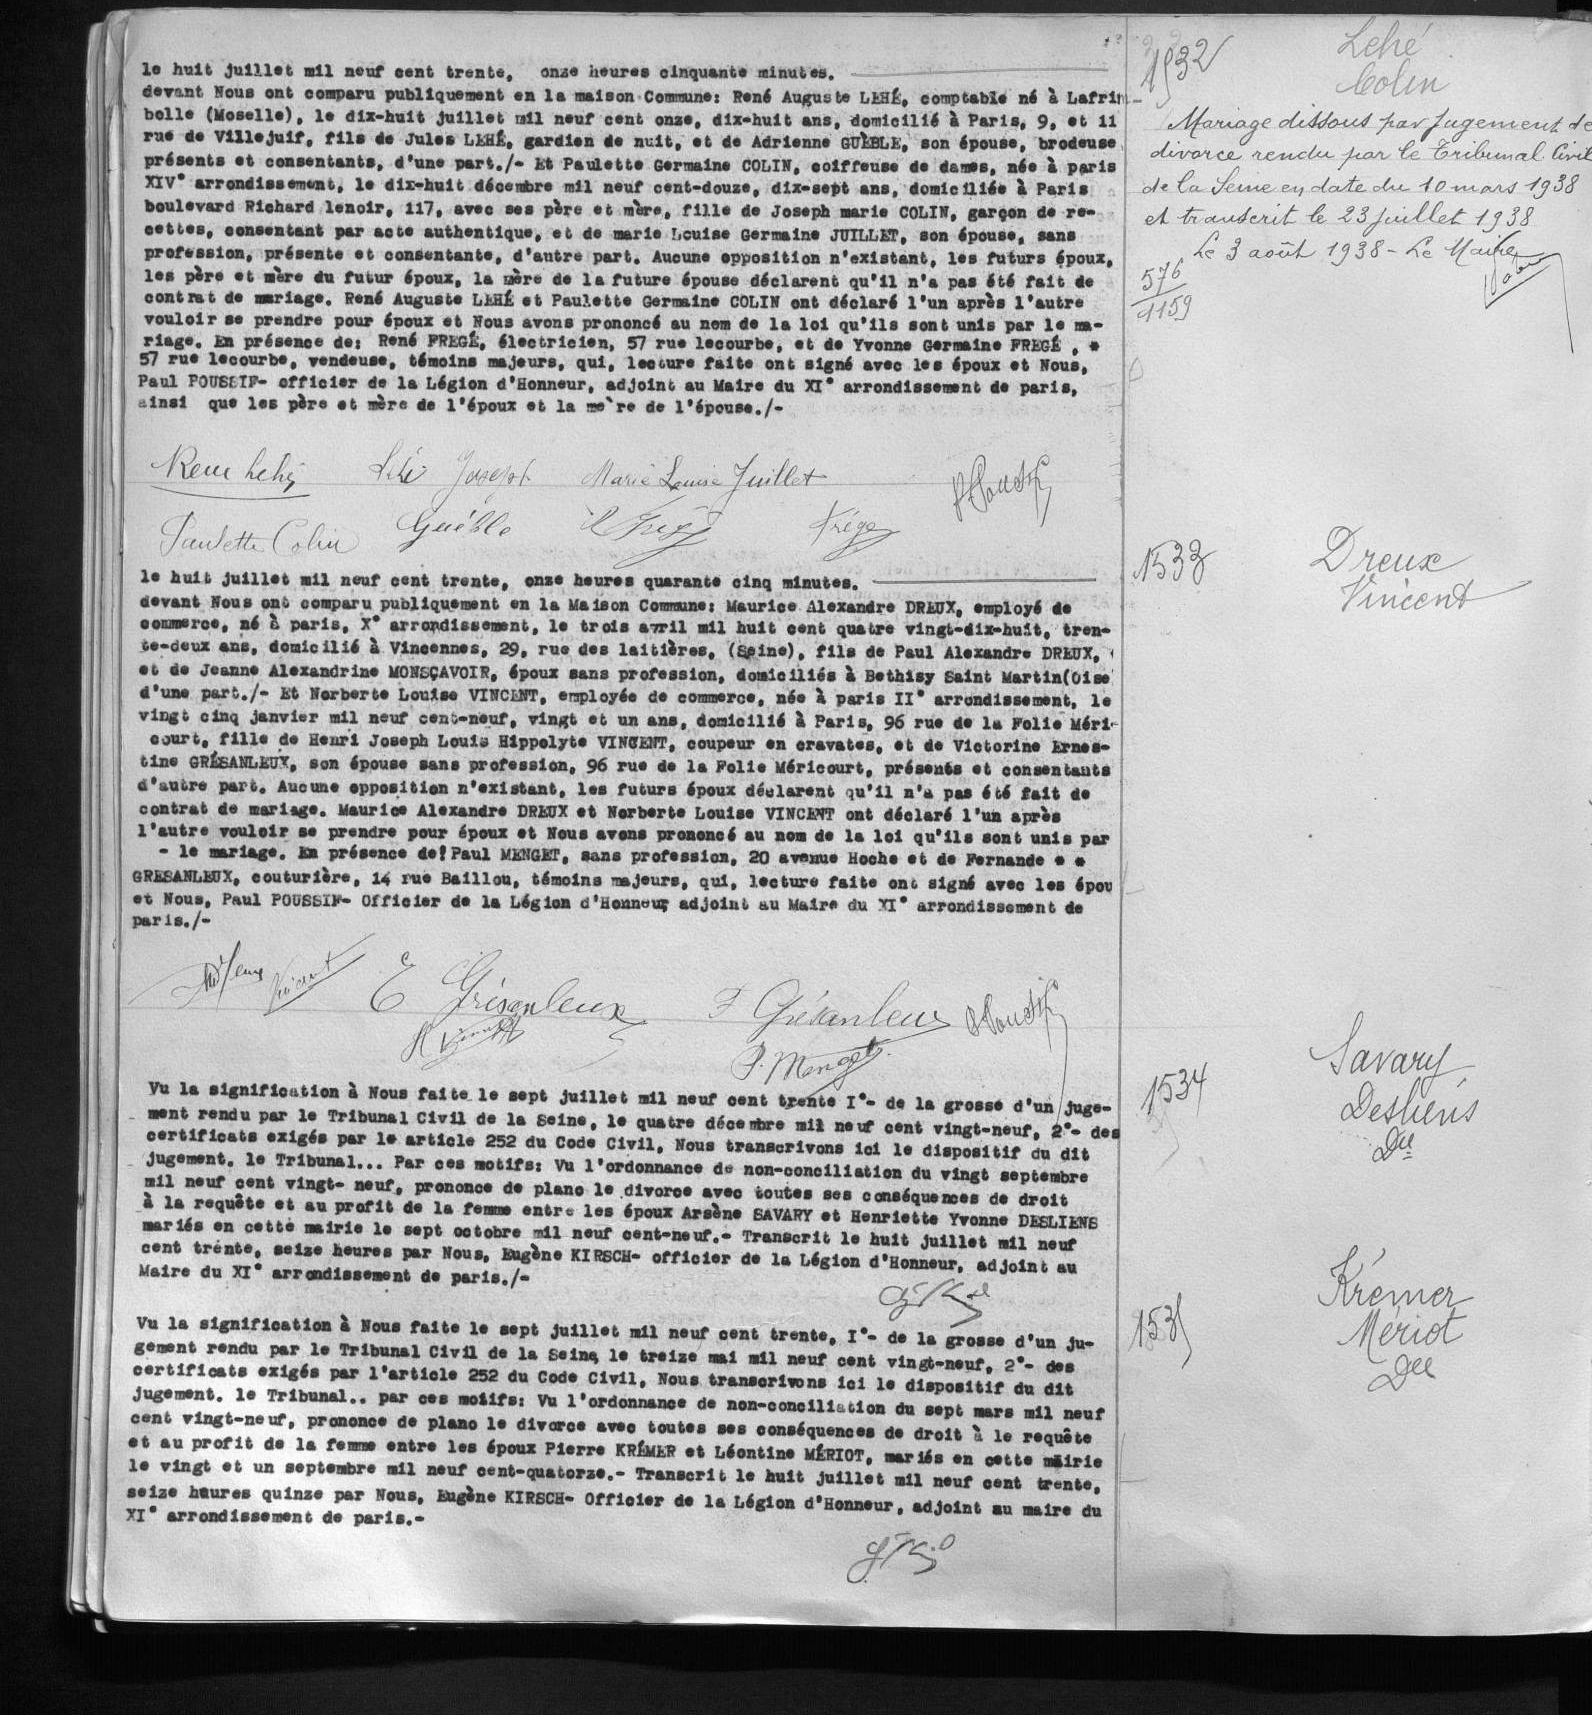le huit juillet mil neuf cent trente, onze heures cinquante minutes .-
devant Nous ont comparu publiquement en la maison commune: René Auguste LEHE, comptable né à Lafrin
belle (Moselle), le dix-huit juillet mil neuf cent onze, dix-huit ans, domicilié à Paris, 9, et 11
rue de Villejuif, fils de Jules LEHE, gardien de nuit, et de Adrienne GUEBLE, son épouse, brodeur
présents et consentants, d'une part ./-Et Paulette Germaine COLIN, coiffeuse de dames, née à paris
XIV ° arrondissement, le dix-huit décembre mil neuf cent-douze, dix-sept ans, domiciliée à Paris
boulevard Richard Lenoir, 117, avec ses père et mère, fille de de Joseph marie COLIN, garçon de re-
cettes, consentant par acte authentique, et de marie Louise Germaine JUILLET, son épouse, sans
profession, présente et consentante, d'autre part. Aucune opposition n'existant, les futrus époux,
les père et mère du futur époux, la mère de la future épouse déclarent qu'il n'a pas été fait de
contrat de mariage. René Auguste LEHE et Paulette Germaine COLIN ont déclaré l'un après l'autre
vouloir se prendre pour époux et Nous avons pronocné au nom de la loi qu'ils sont unis par le ma-
riage. En présence de: René FREGE, électricien, 57 rue lecourbe, et de Yvonne Germaine FREGE, *
57 rue lecourbe, vendeuse, témoins majeurs, qui, lecture faite ont signé avec les époux et Nous,
Paul POUSSIF-officier de la Légion d'Honneur, adjoint au Maire du XI ° arrondissement de paris,
ainsi que les père et mère de l'époux et la mère de l'épouse ./-
le huit juillet mil neuf cent trente, onze heures quarante cinq minutes .-
devant Nous ont comparu publiquement en la Maison Commune: Maurice Alexandre DREUX, employé de
commerce, né à paris, X ° arrondissement, le trois avril mil huit cent quatre vingt-dix-huit, tren-
te-deux ans, domicilié à Vincennes, 29, rue des laitières, (Seine), fils de Paul Alexandre DREUX,
et de Jeanne Alexandrine MONSCAVOIR, époux sans profession, domiciliés à Bethisy Saint Martin (Oise
d'une part ./-Et Noberte Louise VINCENT, employée de commerce, née à paris II ° arrondissement, le
vingt cinq janvier mil neuf cent-neuf, vingt et un ans, domicilié à Paris, 96 rue de la Folie Méri)
court, fille de Henri Joseph Louis Hippolyte VINCENT, coupeur en cravates, et de Victorine Erne-
tine GRESANLEUX, son épouse sans profession, 96 rue de la Folie Méricourt, présents et consentants
d'autre part. Aucune opposition n'existant, les futurs époux déclarent qu'il n'a pas été fait de
contrat de mariage. Maurice Alexandre DREUX et Norbert Louise VINCENT ont déclaré l'un après
l'autre vouloir se prendre pour époux et Nous avons prononcé au nom de la loi qu'ils sont unis par
-le mariage. En présence de: Paul MENGET, sans profession, 20 avenue Hoche et de Fernande **
GRESANLEUX, couturière, 14 rue Baillou, témoins majeurs, qui, lecture faite ont signé avec les épou
et Nous, Paul POUSSIF-Officier de la Légion d'Honneur adjoint au Maire du XI ° arrondissement de
paris ./-
Vu la signification à Nous faite le sept juillet mil neuf cent trente I °-de la grosse d'un juge-
ment rendu par le Tribunal Civil de la Seine, le quatre décembre mil neuf cent vingt-neuf, 2°-des
certificats exigés par l * article 252 du Code Civil, Nous transcrions ici le dispositif du dit
jugement. le Tribunal... Par ces motifs: Vu l'ordonnance de non-conciliation du vingt septembre
mil neuf cent vingt-neuf, prononce de plano le divorce avec toutes ses conséquences de droit
à la requête et au profit de la femme entre les époux Arsène SAVARY et Henriette Yvonne DESLIENS
mariés en cette mairie le sept octobre mil neuf cent-neuf .-Transcrit le huit juillet mil neuf
cent trente, seize heures par Nous, Eugène KIRSCH-officier de la Légion d'Honneur, adjoint au
Maire du XI ° arrondissement de paris ./-
Vu la signification à Nous faite le sept juillet mil neuf cent trente, I °-de la grosse d'un ju-
gement rendu par le Tribunal Civil de la Seine, le treize mai mil neuf cent vingt-neuf, 2°-des
certificats exigés par l'article 252 du Code Civil, Nous transcrivons ici le dispositif du dit
jugement. le Tribunal.. par ces motifs: Vu l'ordonnance de non-conciliation du sept mars mil neuf
cent vingt-neuf, prononce de plano le divorce avec toutes ses conséquences de droit à le requête
et au profit de la femme entre les époux Pierre KREMER et Léontine MERIOT, mariés en cette mairie
le vingt et un septembre mil neuf cent-quatorze .-Transcrit le huit juillet mil neuf cent trente,
seize heures quinze par Nous, Eugène KIRSCH-Officier de la Légion d'Honneur, adjoint au maire du
XI ° arrondissement de paris.-
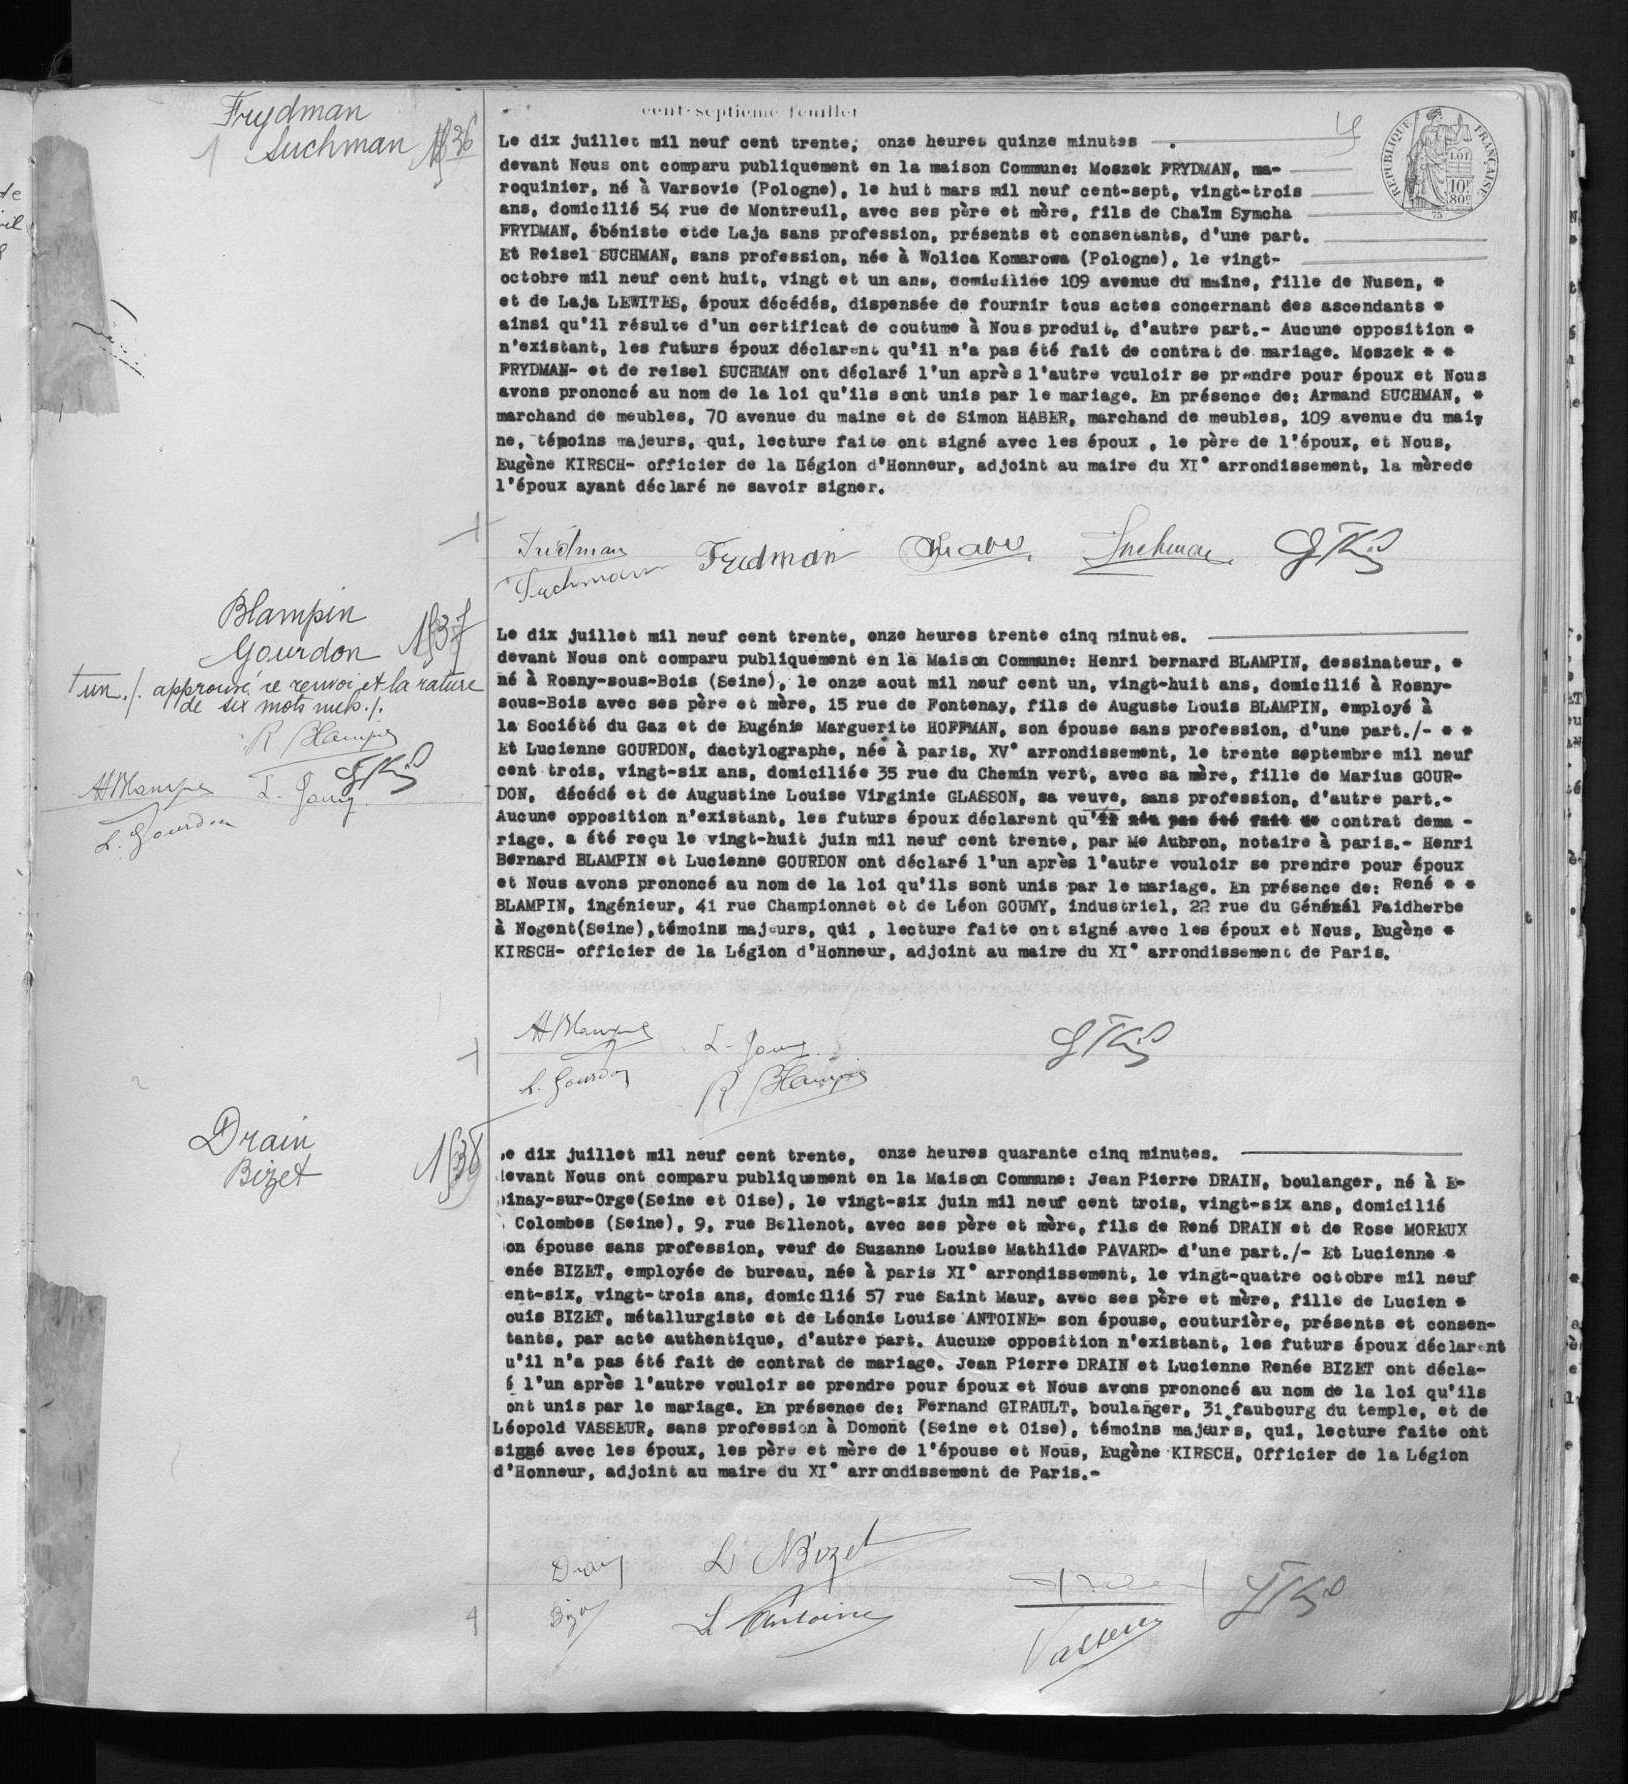Le dix juillet mil neuf cent trente: onze heures quinze minutes -
devant Nous ont comparu publiquement en la maison Commune: Moszek FRYDMAN, ma-
roquinier, né à Varsovie (Pologne), le huit mars mil neuf cent-sept, vingt-trois -
ans, domicilié 54 rue de Montreuil, avec ses père et mère, fils de Chaïm Symcha -
FRYDMAN, ébéniste et de Laja sans profession, présents et consentants, d'une part .-
Et Reisel SUCHMAN, sans profession, née à Wolica Komarowa (Pologne), le vingt -
octobre mil neuf cent huit, vingt et un ans, domiciliée 109 avenue du maine, fille de Nusen, *
et de Laja LEWITES, époux décédés, dispensée de fournir tous actes concernant des ascendants *
ainsi qu'il résulte d'un certificat de coutume à Nous produit, d'autre part .-Aucune opposition *
n'existant, les futurs époux déclarent qu'il n'a pas été fait de contrat de mariage. Moszek **
FRYDMAN-et de reisel SUCHMAN ont déclaré l'un après l'autre vouloir se prendre pour époux et Nous
avons prononcé au nom de la loi qu'ils sont unis par le mariage. En présence de: Armand SUCHMAN, *
marchand de meubles, 70 avenue du maine et de Simon HABER, marchand de meubles, 109 avenue mai,
ne, témoins majeurs, qui, lecture faite ont signé avec les époux, le père de l'époux, et Nous
Eugène KIRSCH-officier de la Dégion d'Honneur, adjoint au maire du XI ° arrondissement, la mère de
l'époux ayant déclaré ne savoir signer.
Le dix juillet mil neuf cent trente, onze heures trente cinq minutes .-
devant Nous ont comparu publiquement en la Maison Commune: Henri bernard BLAMPIN, dessinateur, *
né à Rosny-sous-Bois (Seine), le onze aout mil neuf cent un, vingt-huit ans, domicilié à Rosny-
sous-Bois avec ses père et mère, 15 rue de Fontenay, fils de Auguste Louis BLAMPIN, employé à
la Société du Gaz et de Eugénie Marguerite HOFFMAN, son épouse sans profession, d'une part ./-**
Et Lucienne GOURDON, dactylographe, née à paris, XV ° arrondissement, le trente septembre mil neuf
cent trios, vingt-six ans, domiciliée 35 rue du Chemin vert, avec sa mère, fille de Marius GOUR-
DON, décédé et de Augustine Louise Virginie GLASSON, sa veuve, sans profession, d'autre part .-
Aucune opposition n'existant, les futurs époux déclarent qu'il n'a pas été fait de contrat de ma-
riage. a été recçu le vingt-huit juin mil neuf cent trente, par Me Aubron, notaire à paris .-Henri
Bernard BLAMPIN et Lucienne GOURDON ont déclaré l'un après l'autre vouloir se prendre pour époux
et Nous avons prononcé au nom de la loi qu'ils sont unis par le mariage. En présence de: René **
BLAMPIN, ingénieur, 41 rue Championnet et de Léon GOUMY, industriel, 22 rue du Général Faidherbe
à Nogent (Seine), témoins majeurs, qui, lecture faite ont signé avec les époux et Nous, Eugène *
KIRSCH-officier de la Légion d'Honneur, adjoint au maire du XI ° arrondissement de Paris.
le dix juillet mil neuf cent trente, onze heures quarante cinq minutes .-
evant Nous ont comparu publiquement en la Maison Commune: Jean Pierre DRAIN, boulanger, né à E-
inay-sur-Orge (Seine et Oise), le vingt-six juin mil neuf cent trois, vingt-six ans, domicilié
Colombes (Seine), 9, rue Bellenot, avec ses père et mère, fils de René DRAIN et de Rose MOREUX
on épouse sans profession, veuf de Suzanne Louise Mathilde PAVARD-d'une part ./-Et Lucienne *
enée BIZET, employée de bureau, née à paris XI ° arrondissement, le vingt-quatre octobre mil neuf
en-six, vingt-trois ans, domicilié 57 rue Saint Maur, avec ses père et mère, fille de Lucien*
ouis BIZET, métallurgiste et de Léonie Louise ANTOINE-son épouse, couturière, présents et consen-
tants, par acte authentique, d'autre part. Aucune opposition n'existant, les futurs époux déclarent
qu'il n'a pas été fait de contrat de mariage, Jean Pierre DRAIN et Lucienne Renée BIZET ont décla-
é l'un après l'autre vouloir se prendre pour époux et Nous avons prononcé au nom de la loi qu'ils
ont unis par le mariage. En présence de: Fernand GIRAULT, boulanger, 31 faubourg du temple, et de
Léopold VASSEUR, sans profession à Domont (Seine et Oise), témoins majeurs, qui, lecture faite ont
signé avec les époux, les père et mère de l'épouse de Nous, Eugène KIRSCH, Officier de la Légion
d'Honneur, adjoint au maire du XI ° arrondissement de Paris.-